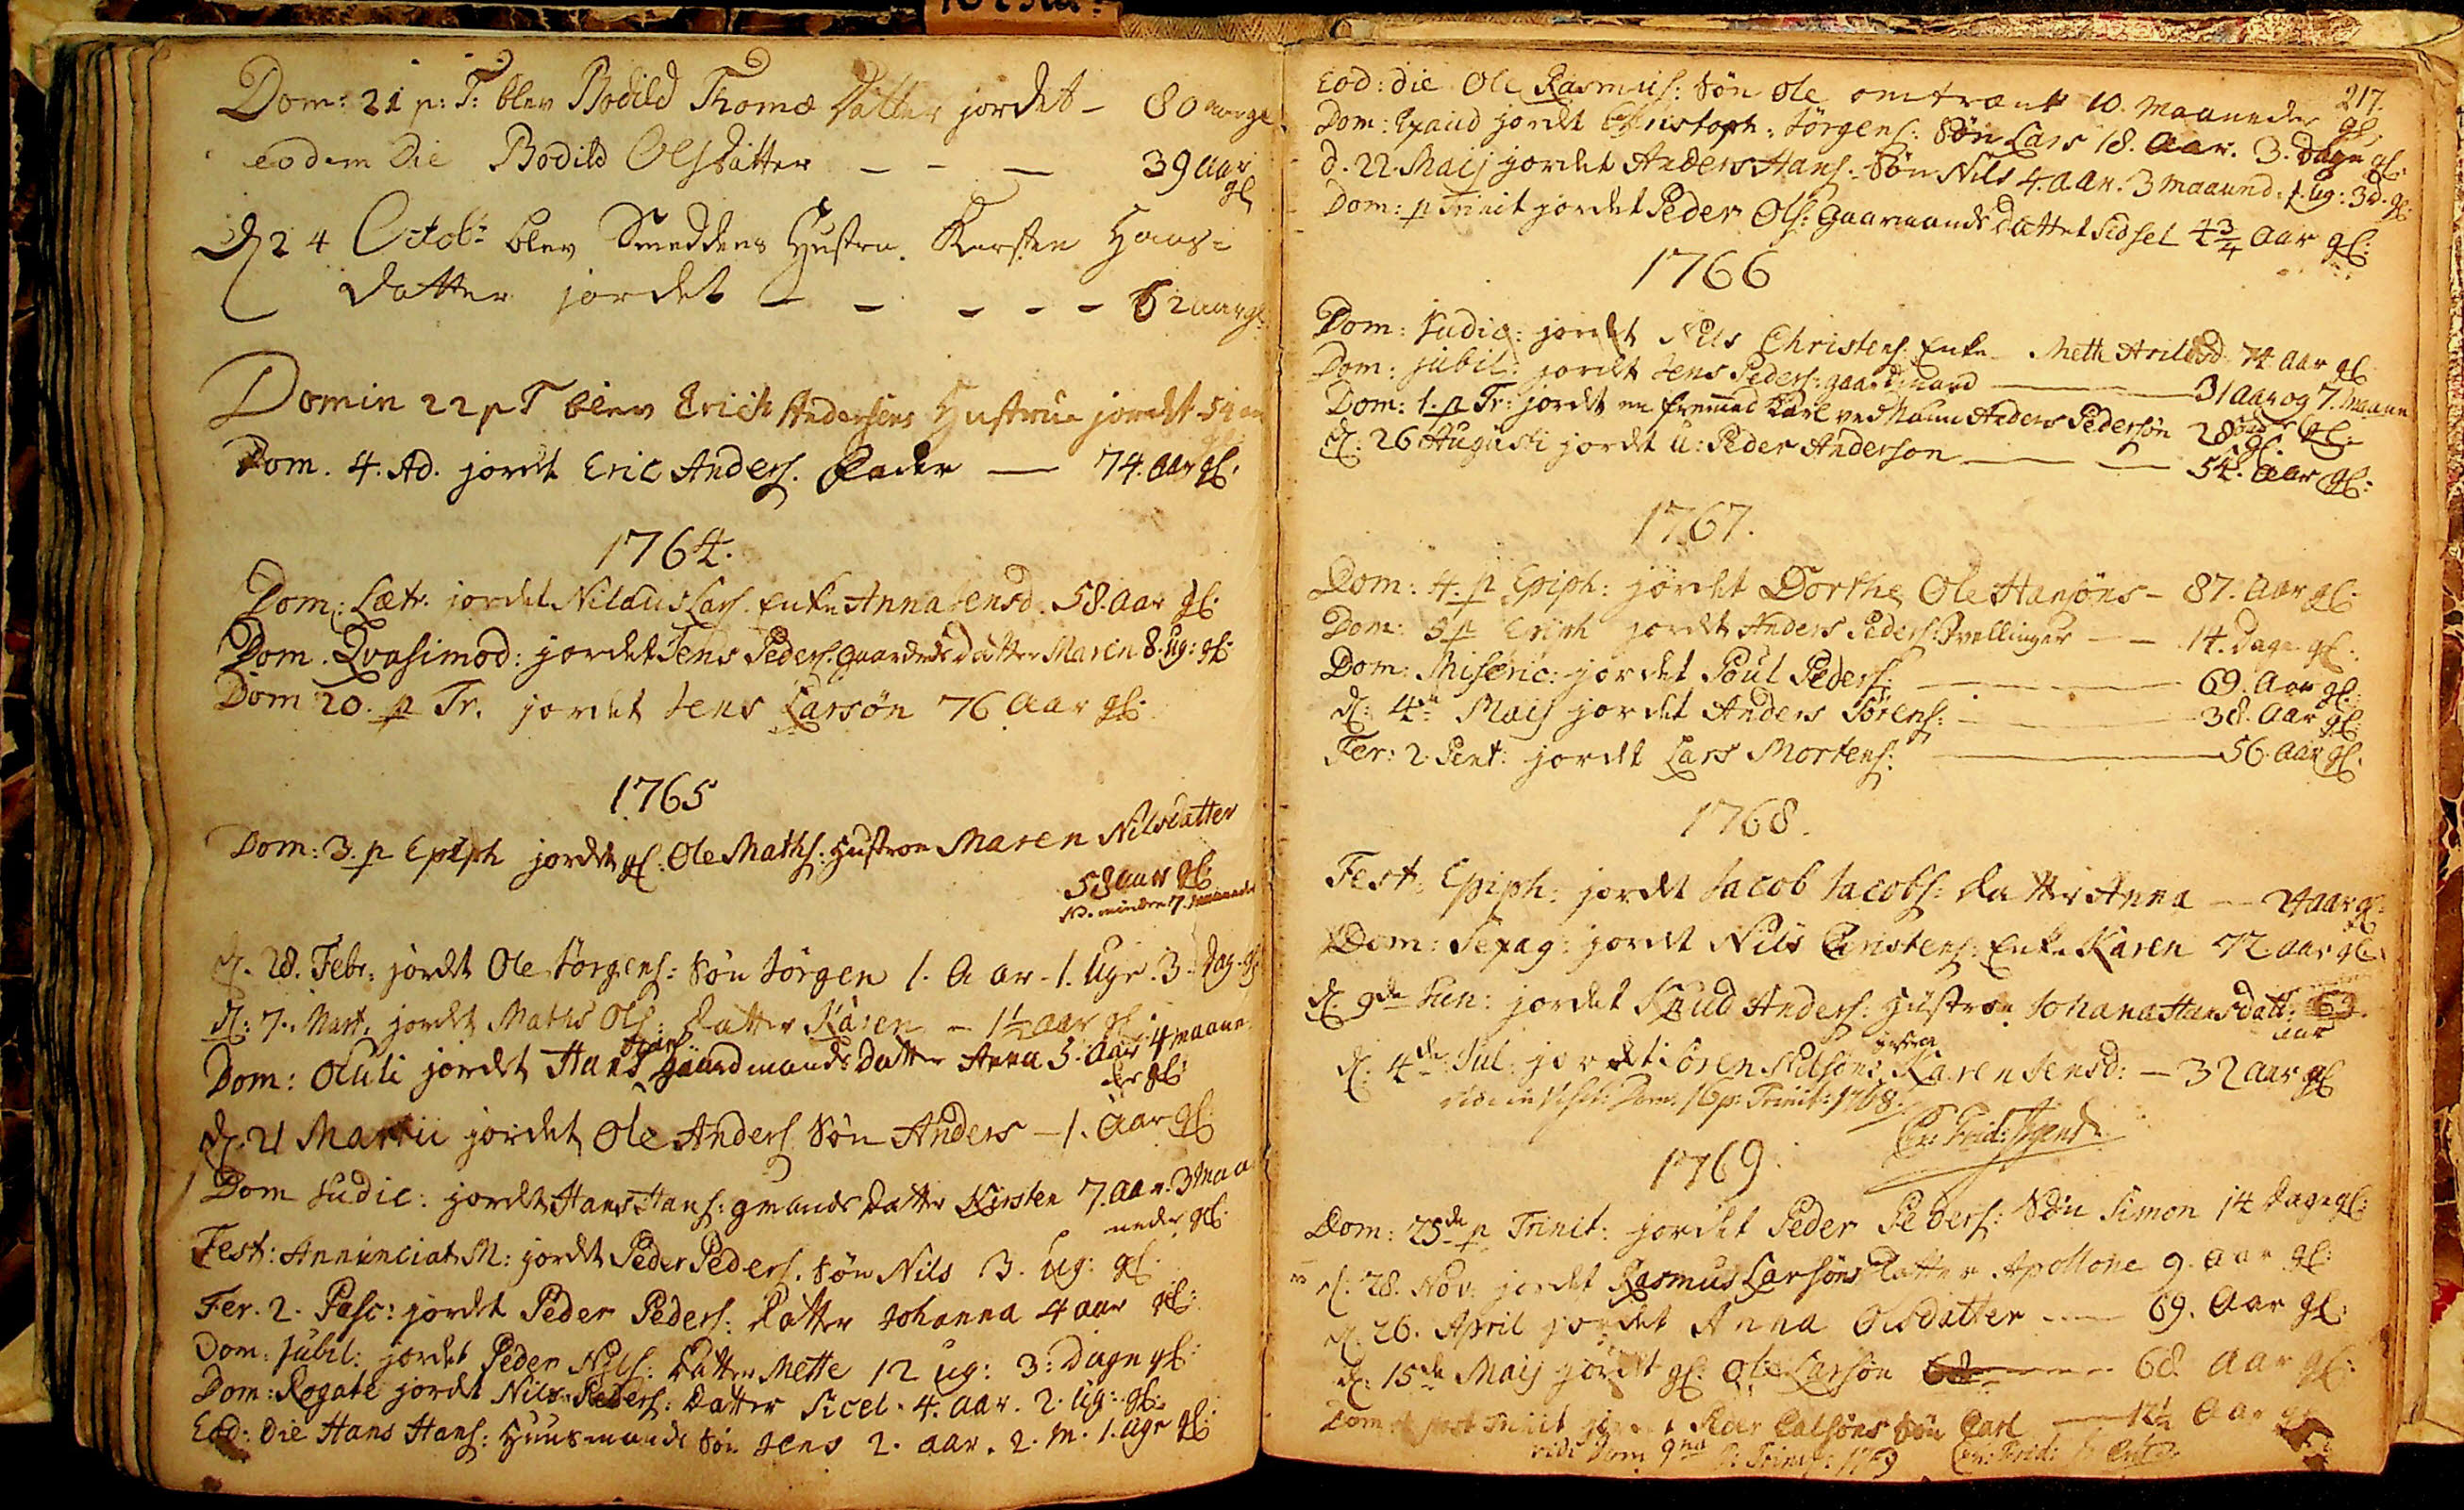Dom: 21 p: T: blev Bodild Thomæ Datter jordet. 80 Aar gl.
eodem die Bodild Olsdatter __ __ __ 39 Aar
gl.
d 24 Octob: blev Smeddens Hustru. Kirsten Hans¬
datter jordet __ __ __ __ __ 62 Aar gl.
Domin 22 p T blev Erich Andersens Hustrue jordet 54 Aar
gl
Dom. 4. Ad: jordet Eric Anders. Fader ___ 74. Aar gl.
1764.
Dom: Lætr. jordet Nilaus Lars. Enke Anna Jensd. 58. Aar gl.
Dom. Qvasimod: jordet Jens Peders. Gaardmds Datter Maren 8.Ug: gl.
Dom: 20. p Tr. jordet Jens Larsøn 76 Aar gl.
1765
Dom: 3. p. Epiph jordet gl: Ole Maths: Hustroe Maren Nielsdatter
53 Aar gl.
NB. minder 7. maaneder
d. 28 Febr: jordet Ole Jørgens: Søn Jørgen 1. Aar. 1. Uge. 3. Dag. gl.
d: 7: Mart: jordet Maths Ols: Datter Karen __ 1½ Aar gl.
Hans
Dom: Oculi jordet Hans Gaardmands Datter Anna 3. Aar 4 maane
der gl:
d. 21 Martii jordet Ole Anders. Søn Anders __ 1. Aar gl:
Dom Judic: jordet Hans Hans: gmands Datter Kirsten 7.Aar. 3 Maa¬
neder gl.
Fest: Annunciat M: jordet Peder Peders. Søn Nils 3. Ug: gl.
Fer. 2. Pasc: jordet Peder Peders: Datter Johanna 4 Aar gl:
Dom: Jubil: jordet Peder Nils: Datter Mette 12 Ug: 3: Dage gl:
Dom: Rogate jordet Nils Peders: Datter Sicel. 4. Aar. 2 Ug: gl:
Eod: Die Hans Hans: Huusmands Søn Jens 2. Aar: 2. M. 1. Uge gl:
217.
Eod: die. Ole Rasmus: Søn Ole omtrænt 10. Maaneder gl.
Dom: Exaud jordt Christoph: Jørgens: Søn Lars 18. Aar. 3. Dage gl.
d. 22 Maij jordet Anders Hans: Søn Niels 4 Aar. 3. Maaned: 1 Ug: 3 d. gl.
Dom: p Trinit jordet Peder Ols: Gaardmands Datter Sidsel 4 3/4 Aar gl:
1766
Dom: Judic: jordet Nils Christens: Enke Mette Arildsd. 74 Aar gl.
Dom: Jubil: jordet Jens Peders: Gaardmand _________ 31 Aar og 7.Maane
der gl:
Aar
Dom: 1. p Tr: jordet en fremmed Karl ved Navn Anders Pedersøn 28 gl:
d: 26 Augusti jordet U: Peder Anderson _____________ ________ 54. Aar gl:
1767.
Dom: 4. p Epiph: jordet Dorthe Ole Hansøns _ 87 Aar gl.
Dom: 5 p Epiph jordet Anders Peders: Tvillinger __ __ 14. Dage gl:
Dom: Miseric: jordet Poul Peders: ___________________ _______ 69. Aar gl:
d: 4de Maij jordet Anders Sørens: ___ ____ __________________  38. Aar gl:
Fer: 2. Pent: jordt Lars Mortens: __________________________ 56. Aar gl:
1768.
Fest Epiph: jordt Jacob Jacobs: datter Anna - - 24 Aar gl:
Dom: Sexag: jordt Nils Christens: Enke Karen 72 Aar gl:
d. 9de Jun: jordet Knud Anders: Hustroe. Johana Hansd: 63.
Aar
Hustroe
d: 4de Jul: jordt Søren Nilsøns Karen Jensd: __ 32 Aar gl.
Vidi in Visit: Dom: 16 p: Trinit: 1768 ./.
Chr: Frid: Irgens
1769.
Dom: 23de p Trinit: jordet Peder Peders: Søn Simon 14 dage gl:
d. 28. Nov: jordet Rasmus Larsøns Datter Apollone 9 Aar gl:
d. 26. April jordet Anna Olsdatter __ __________ 69. Aar gl:
d. 15de Maii jordet gl: Ole Larsøn 68 ___ __________ 68. Aar gl:
Dom: 4 post Trinit jordet Peder Carlsøns Søn Carl ___ 12½ Aar gl
Vidi Dom 9na P: Trinit: 1769. Chr: Frid: Irgens